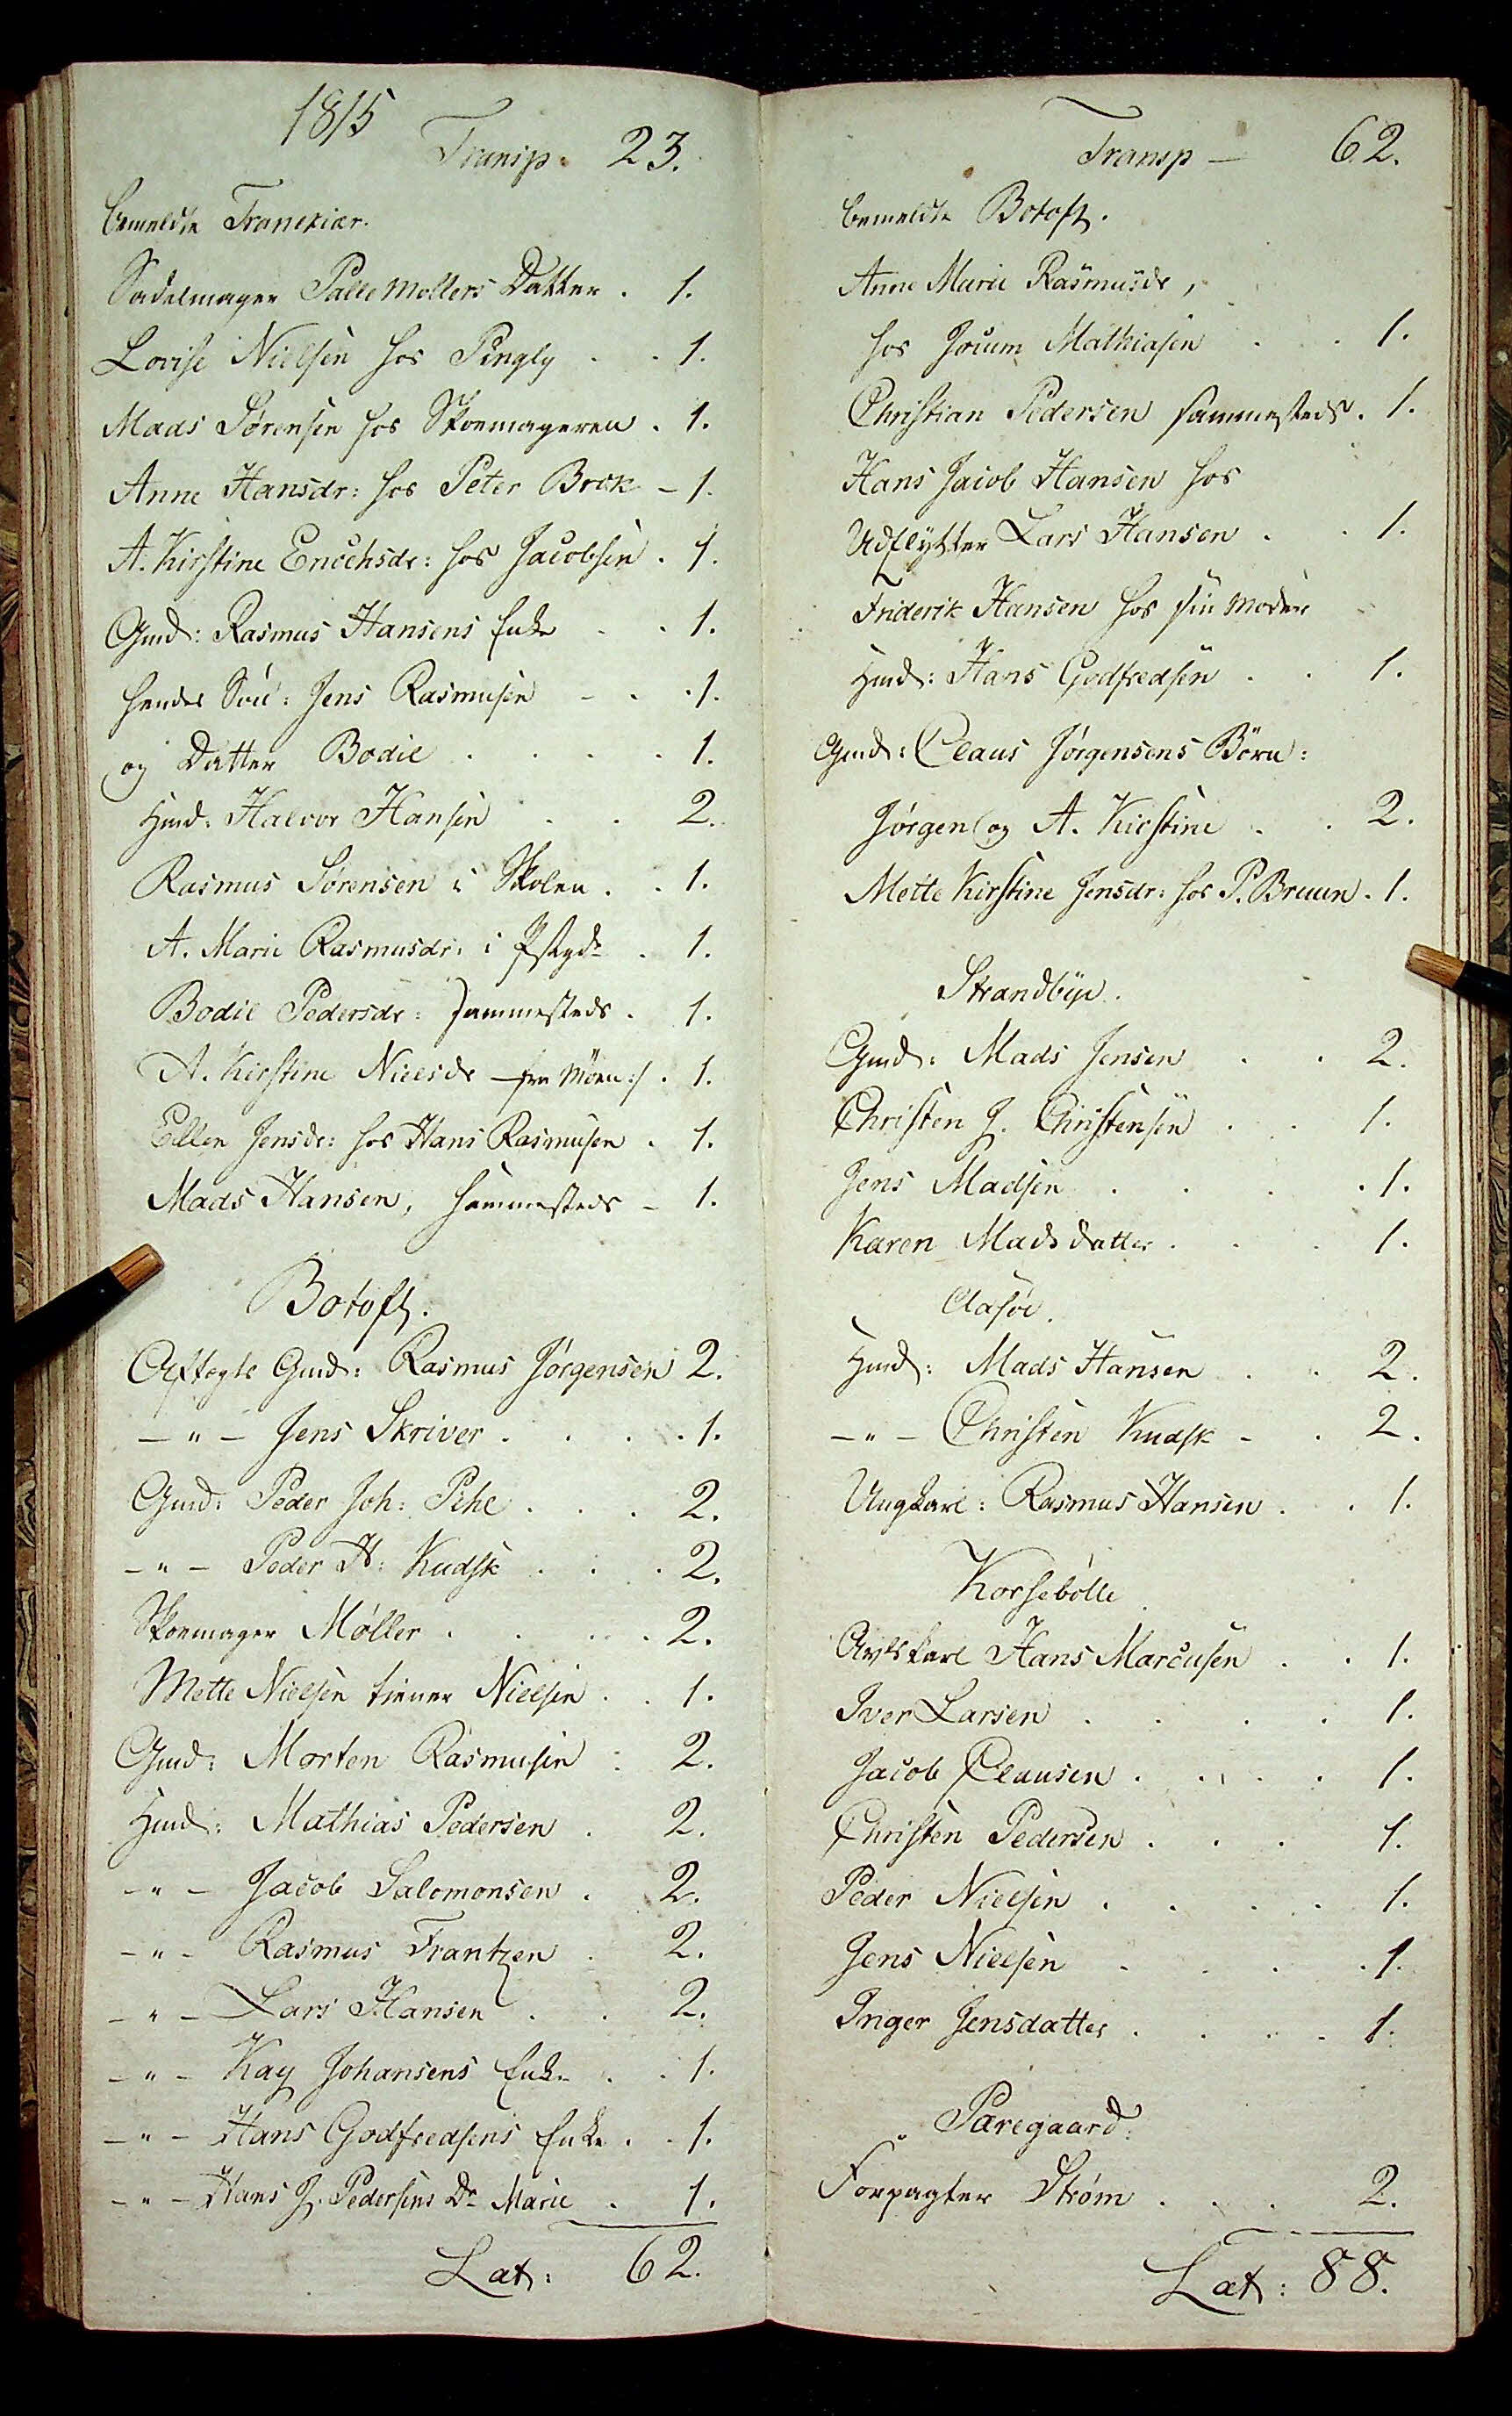1815
Transp: 23.
bemeldte Tranekiær.
Sadelmager Palle Møllers Datter . 1.
Lovise Nielsen hos Pingly . . 1.
Mads Sørensen hos Skoemageren . 1.
Anne Hansdr: hos Peter Brok - 1.
A. Kirstine Enochsdr: hos Jacobsen . 1.
Gmd: Rasmus Hansens Enke . . 1.
hendes Søn: Jens Rasmusen . . . 1.
og Datter Bodil . . . . 1.
Hmd: Halvor Hansen . . 2.
Rasmus Sørensen i Skolen . . 1.
A. Maria Rasmusdr i Pstgdr . 1.
Bodil Pedersdr: sammesteds . 1.
A. Kirstine Nielsdr - fra Møen :/ . 1.
Ellen Jensdr: hos Hans Rasmusen . 1.
Mads Hansen, sammesteds - 1.
Botoft.
Aftægts Gmd: Rasmus Jørgensen 2.
-"- Jens Skriver . . . . 1.
Gmd: Peder Joh: Pihl . . . 2.
-"- Peder H. Kudsk . . . 2.
Skoemager Møller . . . . 2.
Mette Nielsen tiener Nielsen . . 1.
Gmd: Morten Rasmusen : 2.
Hmd: Mathias Pedersen . 2.
-"- Jacob Salomonsen . 2.
-"- Rasmus Frantzen . 2.
-"- Lars Hansen . . 2.
-"- Kay Johansens Enke . . 1.
-"- Hans Godfredsens Enke . . 1.
-"- Hans J. Pedersens Dr Marie . 1.
Lat: 62.
Transp - 62.
bemældte Botoft
Anne Marie Rasmusdr,
hos Jocum Mathiasen . . 1.
Christian Pedersen sammesteds . 1.
Hans Jacob Hansen hos
Udflytter Lars Hansen . . 1.
Friderik Hansen hos sin Moder
Hmd: Hans Godfredsen . . 1.
Gmd: Claus Jørgensens Børn:
Jørgen og A. Kirstine . . 2.
Mette Kirstine Jensdr: hos P. Bruun . 1.
Strandbye.
Gmd: Mads Jensen . . 2.
Christen J. Christensøn . . 1.
Jens Madsen . . . . 1.
Karen Madsdatter . . . 1.
Aasøe.
Hmd: Mads Hansen . . 2.
-"- Christen Kudsk - . 2.
Ungkarl: Rasmus Hansen . . 1.
Korsebølle
Avlskarl Hans Marcusen . . 1.
Peder Larsen . . . . 1.
Jacob Hansen . . . . 1.
Christen Pedersen . . . 1.
Peder Nielsen . . . . 1.
Jens Nielsen . . . . 1.
Inger Jensdatter . . . . 1.
Pæregaard:
Forpagter Strøm . . . 2.
Lat: 88.
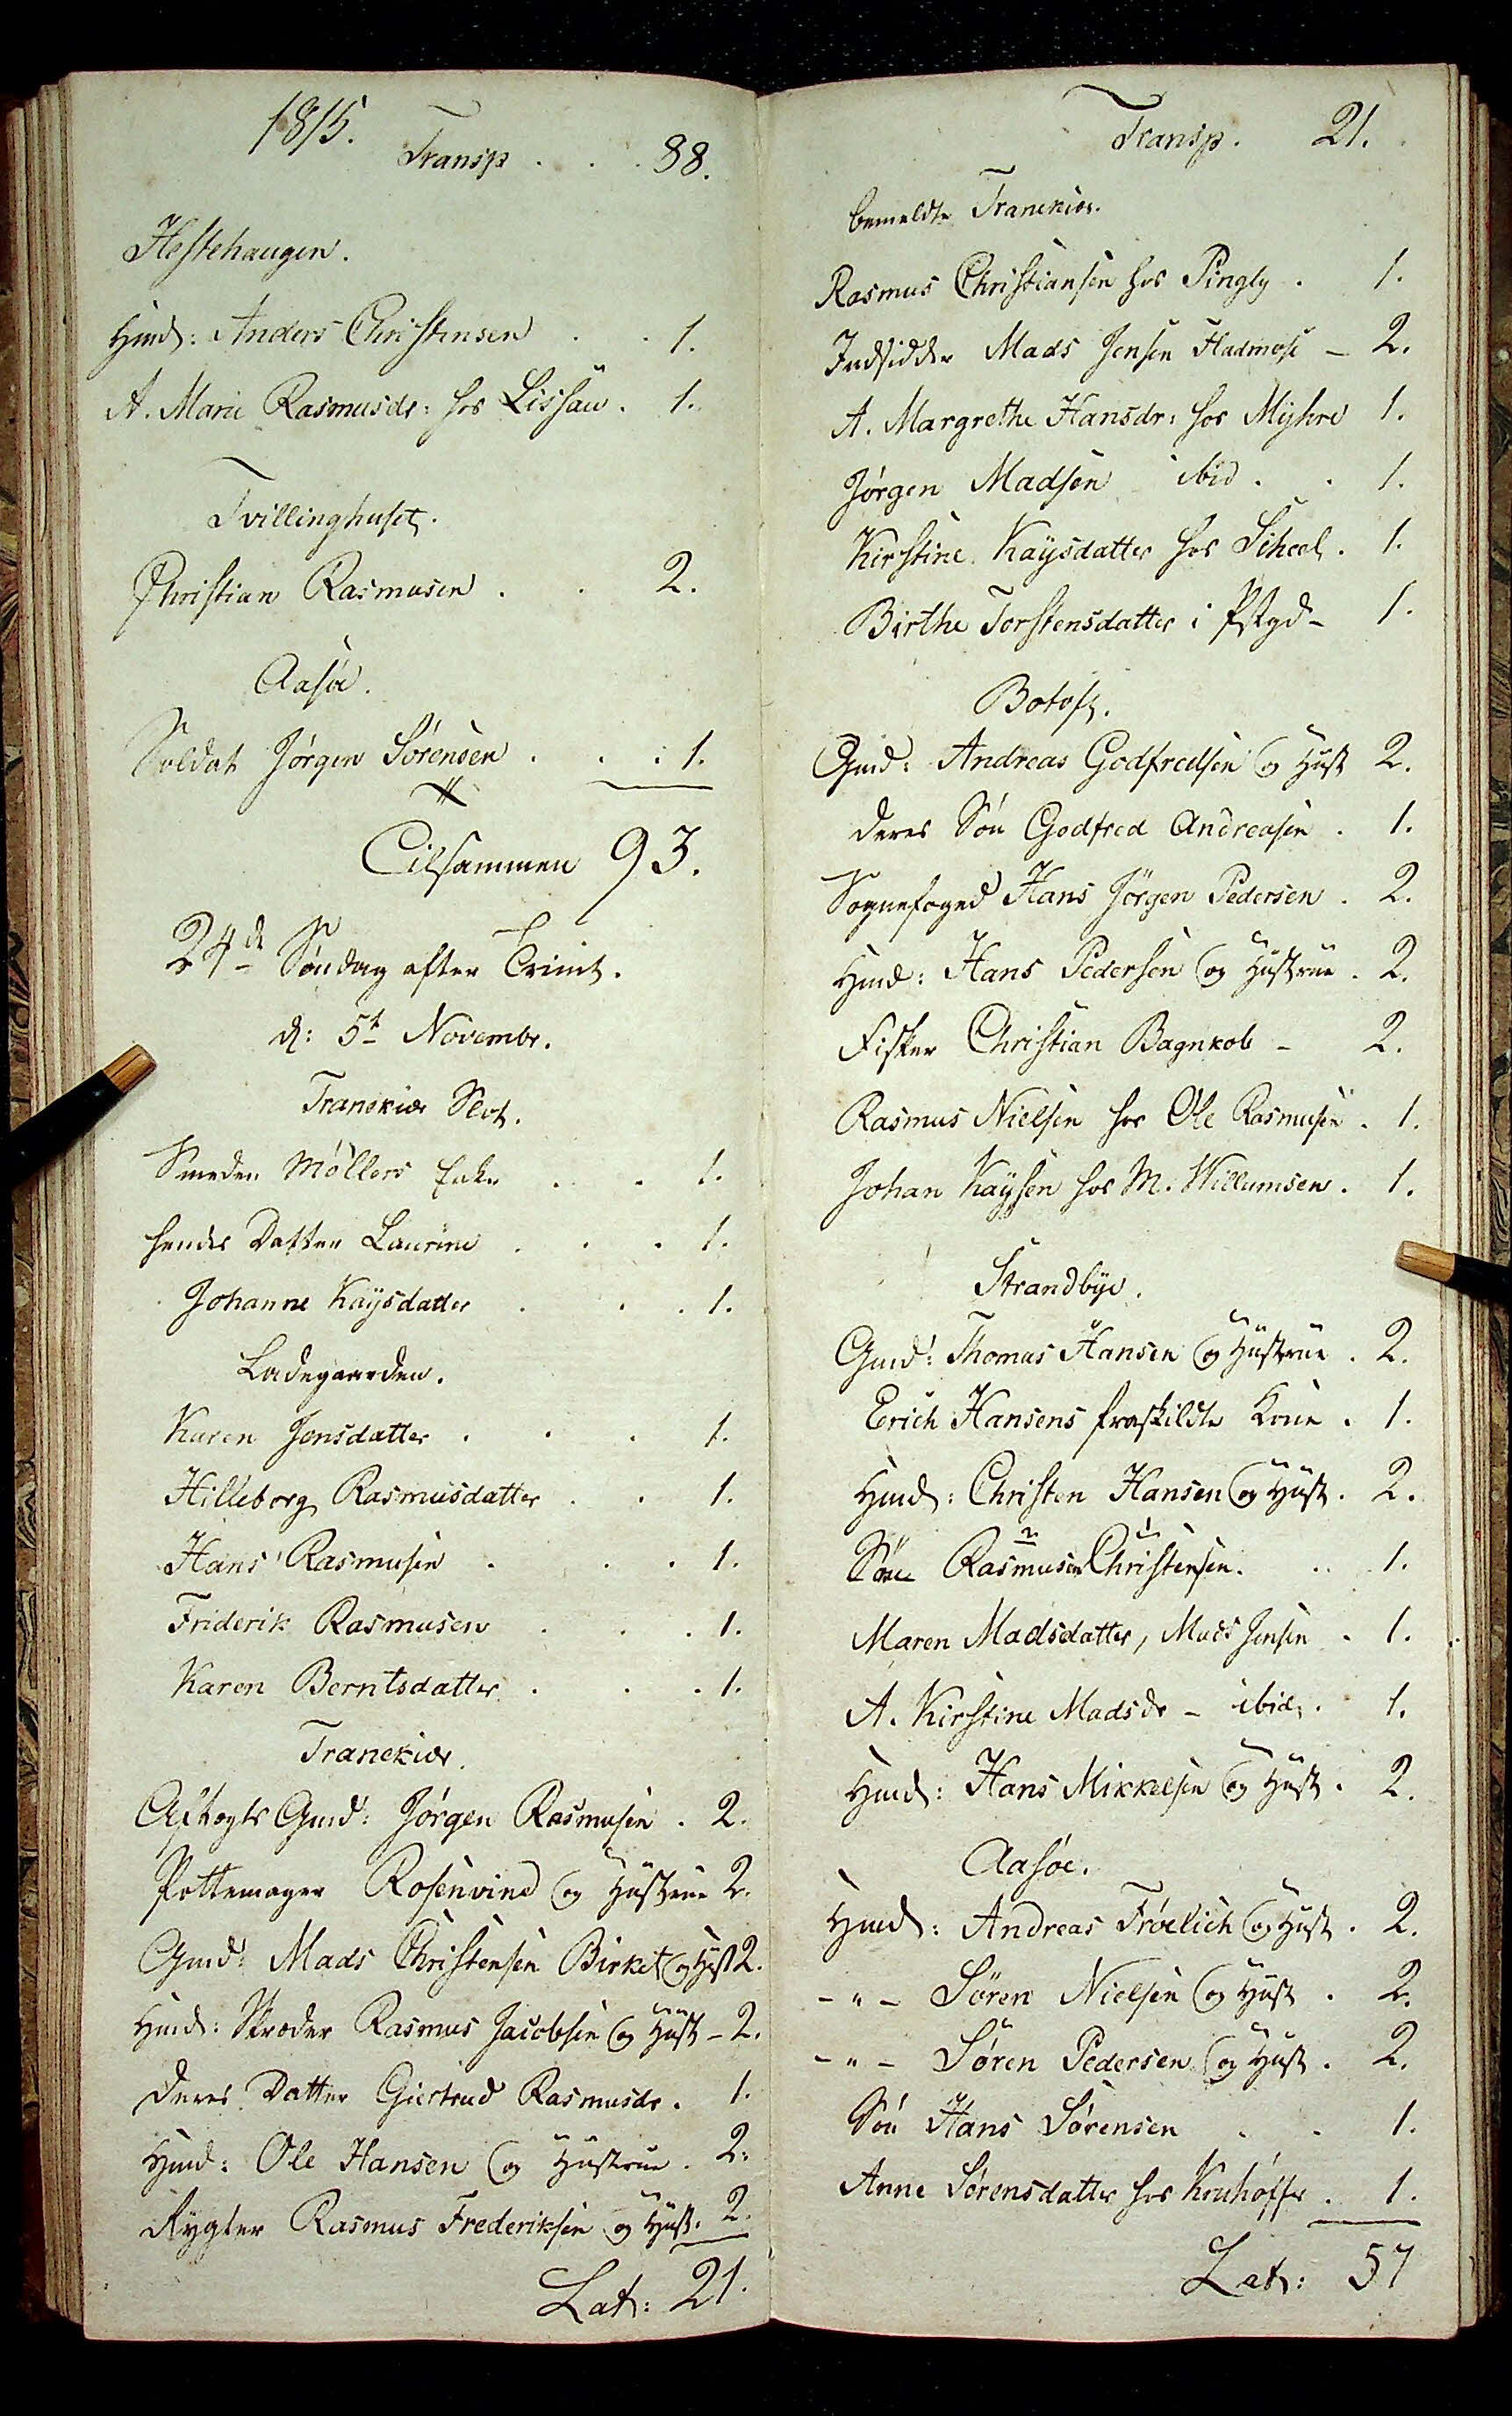1815.
Transp . . . 88.
Hestehaugen.
Hmd: Anders Christensen . . 1.
A. Marie Rasmusdr: hos Lissau . 1.
Tvillinghuset
Christian Rasmusen . . 2.
Aasøe
Soldat Jørgen Sørensen . . . 1.
Tilsammen 93.
24de Søndag efter Trinit.
d: 5te Novembr.
Tranekiær Slot.
Smeden Møllers Enke . . 1.
hendes Datter Laurine . . . 1.
Johanne Kaysdatter . . . 1.
Ladegaarden.
Karen Jensdatter . . . 1.
Hillebog Rasmusdatter . . 1.
Hans Rasmusen . . . 1.
Friderik Rasmusen . . . 1.
Karen Berntsdatter . . . 1.
Tranekiær
Aftægts Gmd: Jørgen Rasmusen . 2.
Pottemager Rosenvind og Hustrue 2.
Gmd: Mads Christensen Birket og Hust 2.
Hmd: Skræder Rasmus Jacobsen og Hust - 2.
deres Datter Giertrud Rasmusdr . 1.
Hmd: Ole Hansen og Hustrue . 2.
Rygter Rasmus Frederiksen og Hust . 2.
Lat: 21.
Transp. 21.
bemeldte Tranekiær.
Rasmus Christiansen hos Pingly . 1.
Indsidder Mads Jensen Hadmose - 2.
A. Margrethe Hansdr: hos Myhre 1.
Jørgen Madsen ibid . . 1.
Kirstine Kaysdatter hos Scheel . 1.
Birthe Torstensdatter i Pstgdr 1.
Botoft.
Gmd. Andreas Godfredsen og Hust 2.
deres Søn Godfred Andreasen . 1.
Sognefoged Hans Jørgen Pedersen . 2.
Hmd: Hans Pedersens og Hustrue . 2.
Fisker Christian Bagnkop - 2.
Rasmus Nielsen hos Ole Rasmusen. 1.
Johan Kaysen hos M. Willumsen . 1.
Strandbye.
Gmd: Thomas Hansen og Hustrue . 2.
Erich Hansens fraskildte Kone . 1.
Hmd: Christen Hansen og Hust . 2.
2
1
Søn Rasmus Christensen . . . 1.
Maren Madsdatter, Mads Jensen . 1.
A. Kirstine Madsdr - ibid . 1.
Hmd: Hans Mikkelsen og Hust . 2.
Aasøe.
Hmd: Andreas Frøelich og Hust . 2.
-"- Søren Nielsen og Hust . 2.
-"- Søren Pedersen og Hust . 2.
Søn Hans Sørensen . .1.
Anne Sørensdatter hos Kruhøffer . 1.
Lat: 57